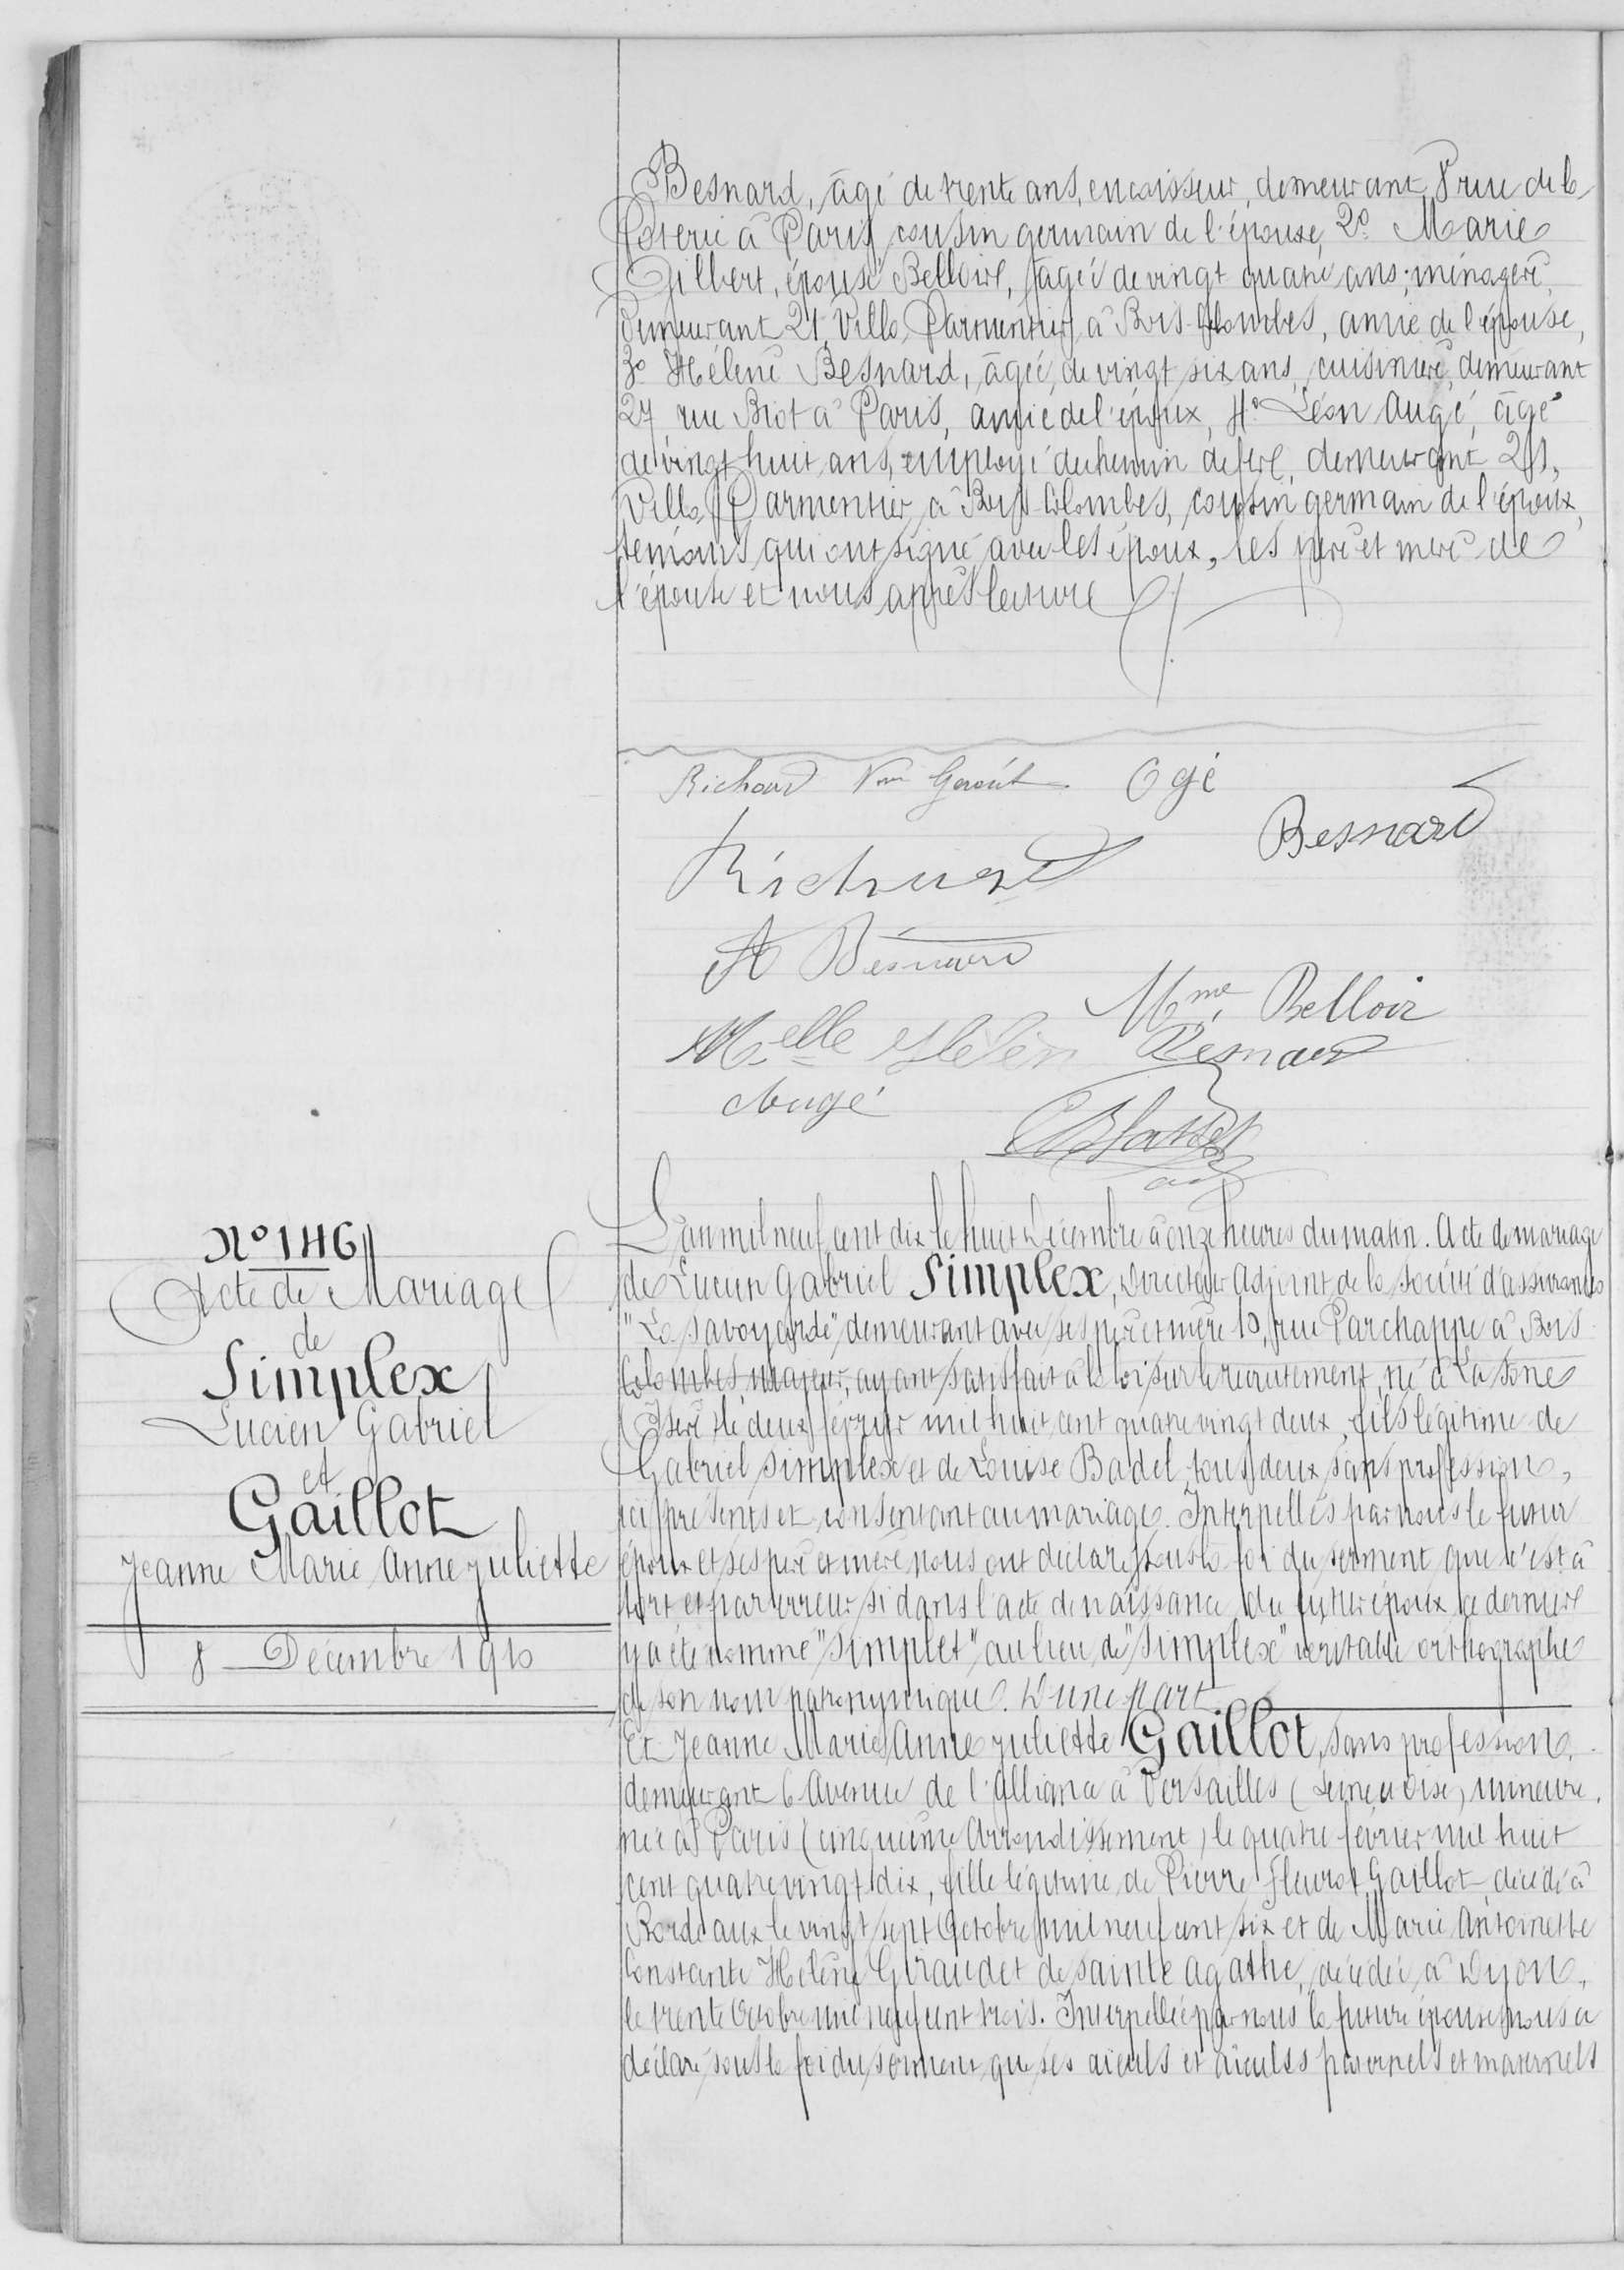Besnard, âgé de trente ans, encaisseur demeurant 8 rue de la
Roserie à Paris, cousin germain de l'épouse, 2° Marie
Gilbert, épouse Belloir, âgée de vingt quatre ans; ménagère
demeurant 21 Villa Parmentier, à Bois-Colombes amie de l'épouse
3° Hélène Besnard, âgée de vingt six ans, cuisinière demeurant
27 rue Biot à Paris, amie de l'époux 4° Léon Augé, âgé
de vingt-huit ans, employé du chemin de fer, demeurant 21
Villa Parmentier, à Bois-Colombes, cousin germain de l'époux,
témoins qui ont signé avec les époux, les père et mère de
l'épouse et nous après lecture./.
N° 146
Acte de Mariage
de
Simplex
Lucien Gabriel
et
Gaillot
Jeanne Marie Anne Juliette
8 Décembre 1910
L'an mil neuf cent dix le huit décembre à onze heures du matin. Acte de mariage
de Lucien Gabriel Simplex, Directeur Adjoint de la société d'assurances
"la savoyarde" demeurant avec ses père et mère 10, rue Parchappe à Bois
Colombes, majeur, ayant satisfait à la loi sur le recrutement né à la Sones
(Isère) le deux février mil huit cent quatre vingt deux, fils légitime de
Gabriel Simplex et de Louise Badel, tous deux sans profession
ici présents et consentant au mariage. Interpellés par nous le futur
époux et ses père et mère nous ont déclaré sous la foi du serment que c'est à
tord et par erreur si dans l'acte de naissance du futur époux ce dernier
y a été nommé "simplet" au lieu de "simplex" véritable orthographe
de son nom patronymique. D'une part -
Et Jeanne Marie Anne Juliette Gaillot, sans professions,
demeurant 6 avenue de l'Alliance à Versailles (Seine et Oise) mineure,
née à Paris (cinquième arrondissement) le quatre février mil huit
cent quatre vingt-dix, fille légitime de Pierre, Fleurot Gaillot, décédé à
Bordeaux le vingt sept octobre mil neuf cent six et de Marie Antoinette
Constance Hélène Giraude de Sainte Agathe, décédée, à Dijon,
le trente octobre mil neuf cent trois. Interpellé par nous la future épouse nous a
déclaré sous la foi du serment que ses aïeuls et aïeules paternels et maternels
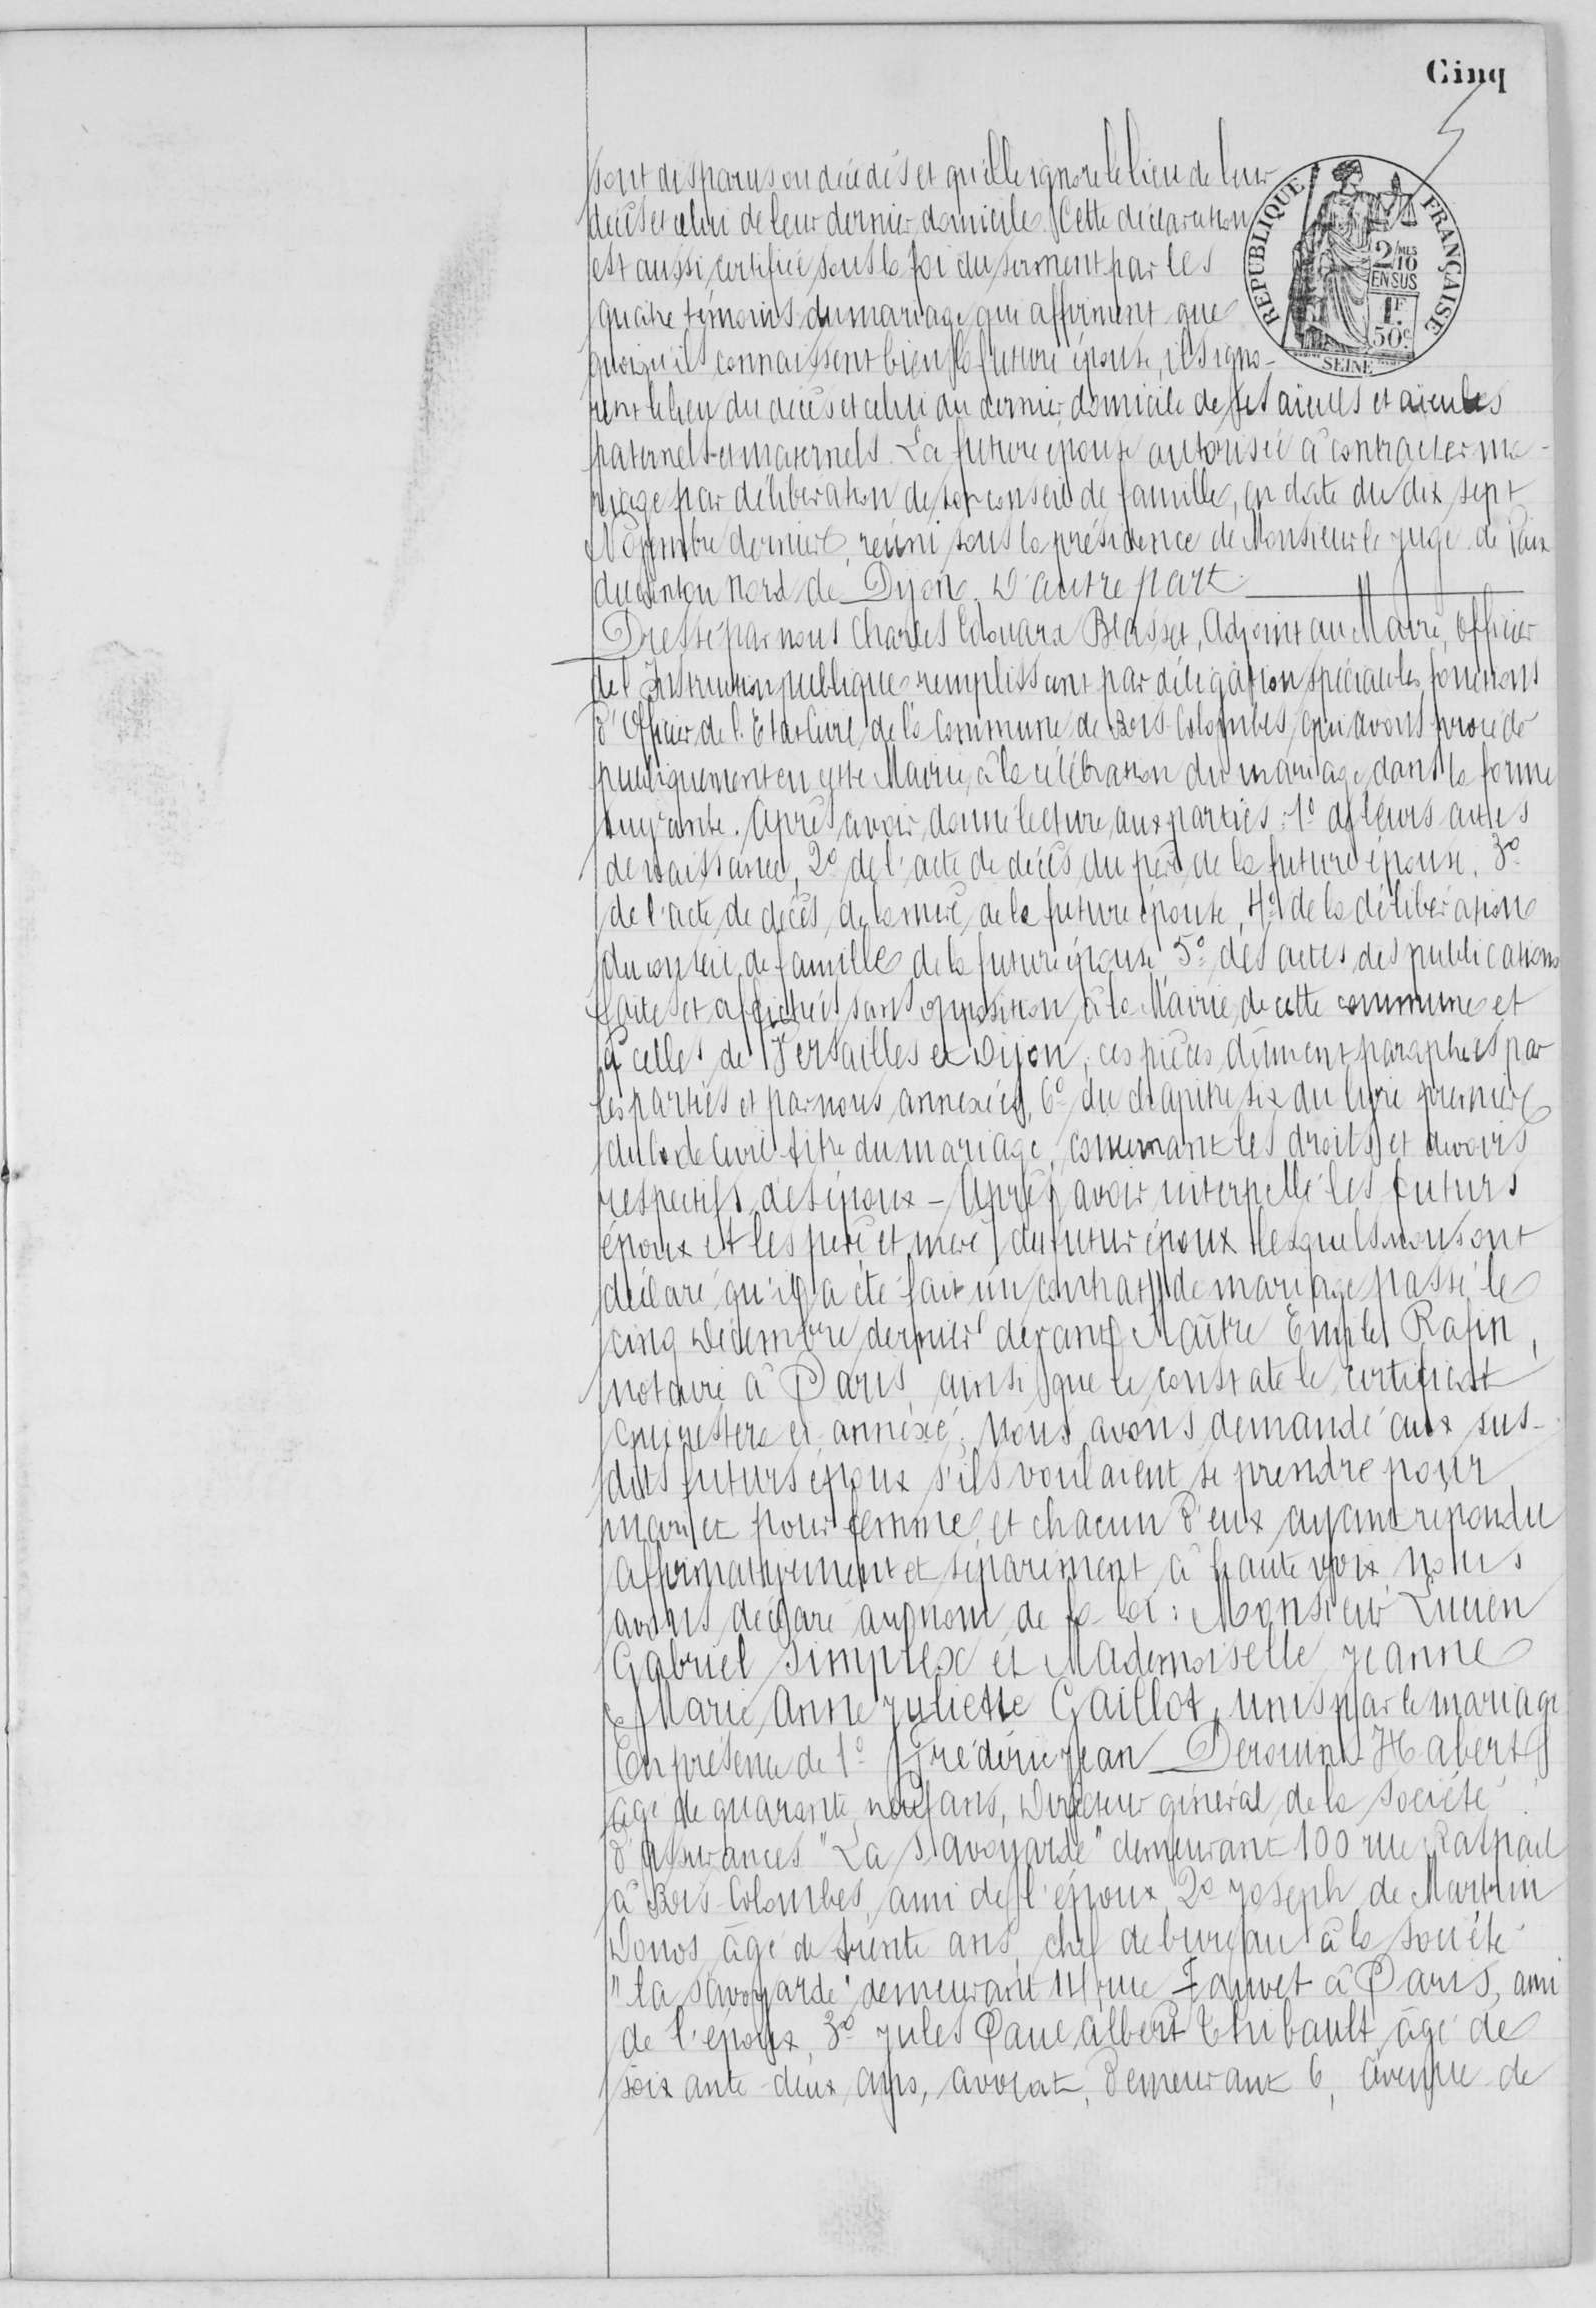sont disparus ou décédés et qu'elle ignore le lieu de leur
décès celui de leur dernier domicile. Cette déclaration
est aussi certifiée sous la foi du serment par les
quatre témoins du mariage qui affirment que
quoiqu'il connaissent bien la future épouse, ils igno-
rent le lieu du décès et celui du dernier domicile de ses aïeuls et aïeules
paternels et maternels. La future épouse autorisée à contracter ma-
riage par délibération de son conseil de famille, en date du dix sept
Novembre dernier, réuni sous la présidence de Monsieur le juge de Paix
du Canton Nord de Dijon D'Autre Part.-
Dressé par nous Charles Edouard Blasset, Adjoint au Marie, Officier
de l'Institution Publique remplissant par délégation spéciales fonctions
d'Officier de l'Etat Civil de la Commune de Bois-Colombes qui avons procédé
publiquement en cette Mairie, à la célébration du mariage dans la form
suivante. Après avoir donné lecture aux parties: 1° de leurs actes
de naissance 2° de l'acte de décès du père de la future épouse, 3°
de l'acte de décès de la mère de la future épouse 4° de la délibération
du conseil de famille de la future épouse 5° des acte des publications
faite et affichées sans opposition à la Mairie de cette commune et
à celle de Versailles et Dijon, ces pièces dûment paraphés par
les parties et par nous, annexées, 6° du chapitre six du livre premier
du Code Civil titre du mariage, concernant les droits et devoirs
respectifs des époux-Après avoir interpellé les futurs
époux et les père et mère du futur époux lesquels nous ont
déclaré qu'il a été fait un contrat de mariage passé le
cinq décembre dernier devant Maitre Emile Rabin
notaire à Paris ainsi que le constat et le certificat
cuistere et annéxé. Nous avons demandé aux sus-
dits futurs époux s'ils voulaient se prendre pour
Mari et pour femme et chacun d'eux ayant répondu
affirmativement et séparément à haute voix nous
avons déclaré au nom de la loi: Monsieur Lucien
Gabriel simplex et Mademoiselle, Jeanne
Marie Anne Juliette Gaillot, unis par le mariage
en présence de 1°: Frédéric Jean Deroin Habert
agé de quarante neuf ans, Directeur général de la société
d'assurances "La Savoyarde" demeurant 100 rue Raspail
à Bois Colombes, ami de l'époux 2° Joseph de Martin
Donos âgé de trente ans, chef de bureau à la société
"la Savoyarde" demeurant 14 rue Fauvet à Paris, ami
de l'époux 3° Jules Paul Albert Thibault, agé de
soixante-deux ans, avocat, demeurant 6, Avenue de